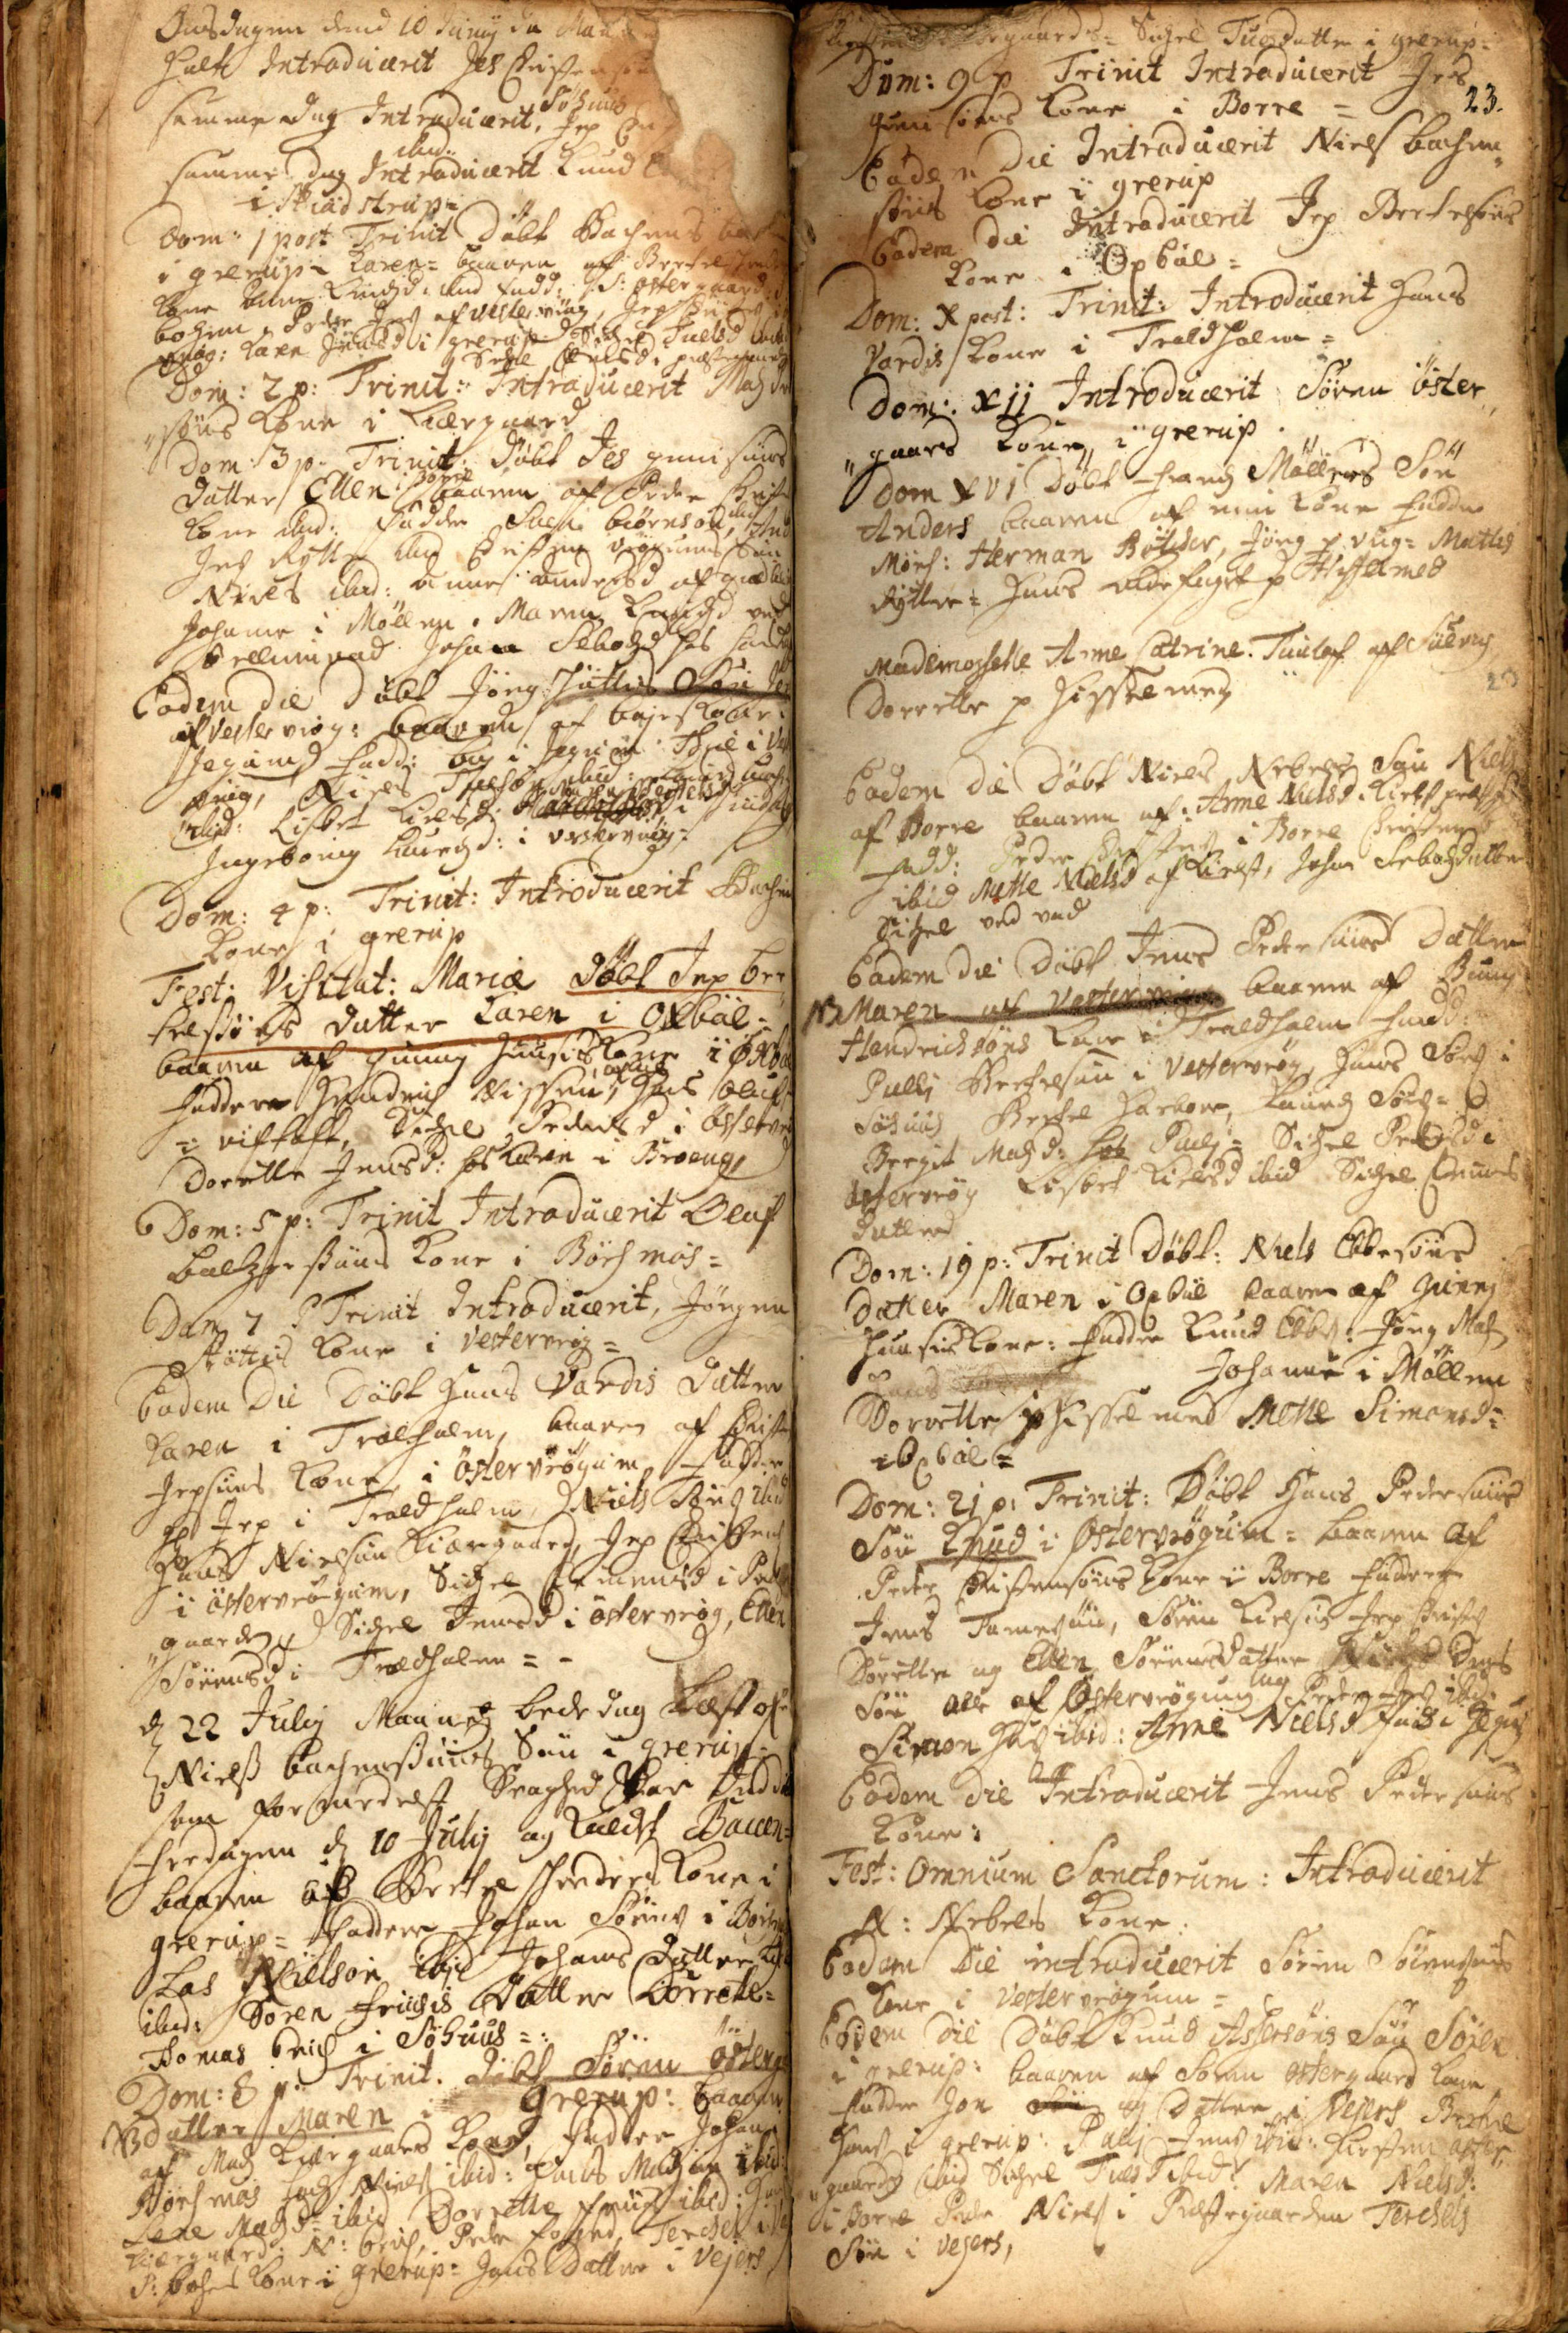Onsdagen dend 10 Junij ## ##
## Introducerit Jes Christen ##
Søhuus
Samme Dag Introduceret Jep ##
ibid:
Samme Dag Introduceiet Knud ##
i Skiødstrup=
Dom: 1 post Trinit Døbt Bachen S##
i Grerup Karen= baaren af Bertel ##
Kone Anne Knudsd ibid Fadd: S. Østergaard##
bachen  Peder Jens af vester vrøg, Jep Christens ##
vrøg: Karen J##d i Grerup: ## Sidsel Tuelsd ##
Sidsel ##elsd i Præstegaard
Dom: 2 p: Trinit. Introducerit Madz Pe
søns Kone i Kiærgaard
Dom: 3 p.Trinit. døbt Jes gunisøns
Datter Ellen i Borre, baaren af Peder Christen
##
Kone ibid: Faddere Sven biørnsøn, And
Jes Rytter ibid Christen Vrøgums Søn
Niels ibid: Anne Andersd af Giedbierg
Johanne i Møllen, Maren Lauridzd ved
Billumvad Johan Sebosd hos ##
Eodem Die døbt Jørg Skøttis Søn Jes
af Vestervrøg: baaren af Bojes Kone ##
Jegum. Fadd: Boj i Jegum, Thue i Vest
vrøg Niels Tuesøn ibid: Lauridz Bach:
##
ibid: Lisbet Kielsd ## i Tudal
Ingeborig Laurdzd: i Vestervrøg:
Dom: 4 p: Trinit: Introducerit Bachens
Kone i Grerup
Fest: Visitat: Mariæ døbt Jep Ber¬
telsøns Datter Karen i Oxbøl=
baaren af Gunnj Juustis Kone i Oxbøl.
Faddere: Hendrich Nissen i Oxbøl, Hans Olufs
i Viftofte, Sidsel Pedersd i Østervrøg
Dorette Jensd: hos Karen i Broeng
Dom: 5 p:Trinit Introducerit Oluf
Balzersøns Kone i Børsmos=
Dom 7 P Trinit Introducerit,  Jørgen
Skøttis Kone i Vestervrøg=
Eodem Die Døbt Hans Vardis Datter
Karen i Troldholm, baaren af Christen
Jepsøns Kone i Østervrøgum - Faddere
gl Jep i Troldholm, Niels ## ibid
Hans Nielsøn Kiærgaard, Jep Christens
i Østervrøgum, Sidzel Clemmensd i Præ
gaarden, Sidzel Jensd i Østervrøg, Ellen
Sørensd i Troldholm= -
d 22 Julij Maanedz Bededag Læst of
Niels Bachensøns Søn i Grerup,
som formedelst Svaghed var Ind##
Fredagen d 10 Julij og kaldet Baccen:
baaren af Bertel Shreders Kone i
Grerup= Faddere Johan Sørens i Bør##
Las Nielsøn ibid, Johans Datter ##
ibid: Søren Friisis Datter Dorrette=
Thomas Brich i Søhuus= :
Dom: 8 p. Trinit. døbt Søren Østerg
s Datter Maren i Grerup: baaren
af Madz Kiærgaards Kone, Fadder Johan i
Børsmos Las Nielsen ibid: Hans Madzøn ibid:
Lene Mdzd= ibid Dorrette Friis ibid: G##
Kiærgaard: N. Brich, Peder Foged, Terchel i V##
##: Bah## Kone i Grerup, Jens Datter i Vejers
23
23
##gaards= Sidzel Tuesdatter i Grerup.
Dom: 9 p Trinit Introducerit Jes
Gunnisøns Kone i Borre=
Eodem Die Introducerit Niels Bachen¬
søns Kone i Grerup
Eoden Die Introducerit Jep Bertelsøns
Kone i Oxbøl=
Dom: X post: Trinit: Introducerit Hans
Vardis Kone i Troldholm=
Dom: XII Introducerit Søren Øster¬
gaards Kone i Grerup.
Dom XVI Døbt Frandz Møllers Søn
Anders baaren af min Kone. Faddere
Møns: Herman Bøtcher, Jørg ##= Mathis
Rytter = Hans Ladefoget p Hisselmed
Mademochelle Anne Catrine. Taulof af Søevig
Dorrette p Hisselmed,
Eodem Die Døbt Niels Nebels Søn Niels
af Borre baaren af: Anne Nielsd. Kielst præsteg
Fadd: Peder Christens i Borre, Christen ##
ibid Mette Nielsd af Kielst, Johan Sebæsdatter
Sidzel ved vad
Eodem Die Døbt Jens Pedersøns Datter
## Maren af Vestervrøg baaren af Gunnj
Hendrichsøns Kone i Troldholm Fadd:
Palle Bertelsøn i Vestervrøg, Hans Sørens i
Søhuus Bertel Harboes, Lauridz Sørens
Bergit Madzd: hos Pallj= Sidzel Pedersd i
Østervrøg Lisbet Kielsd ibid Sidzel Clemmens
datter
Dom: 19 p: Trinit Døbt: Niels Ebbesøns
Datter Maren i Oxbøl baaren af Gunnj
Huusis Kone: Faddere Knud Ebbes:  Jørgen Mads
Hans ##
Johanne i Møllen
Dorrette p Hisselmed, Mette Simonsd:
i Oxbøl=
Dom: 21 p: Trinit: Døbt Hans Pedersøns
Søn Knud i Østervrøgum= baaren af
Peder Christensøns Kone i Borre. Faddere
Jens Tamesøn, Søren Kielsøn Jep Christens
Dorrette og Ellen Sørensdatter Niels ##
Søn alle af Østervrøgum og Peder Jess ibid
Simon H## ibid: Anne Nielsd J## i Jegum
Eodem Die Introducerit Jens Pedersøns
Kone:
Fest. Omnium Sanctorum: Introducerit
N: Nebels Kone:
Eodem Die Introducerit Søren Sørensøns
Kone i Vestervrøgum=
Eodem Die Døbt Knud Assersøns Søn Søren
i Grerup: baaren af Soren Østergaard Kone,
Fadder Jon ## ## Datter i Vejers, Bertel
Hans i Grerup:  Pallj Jens ibid: Kiersten Øster
gaards ibid, Sidzel ## ##: Maren Nielsd:
i Borre Peder Niels i Præstegaarden, Terchels
Søn i Vejers,
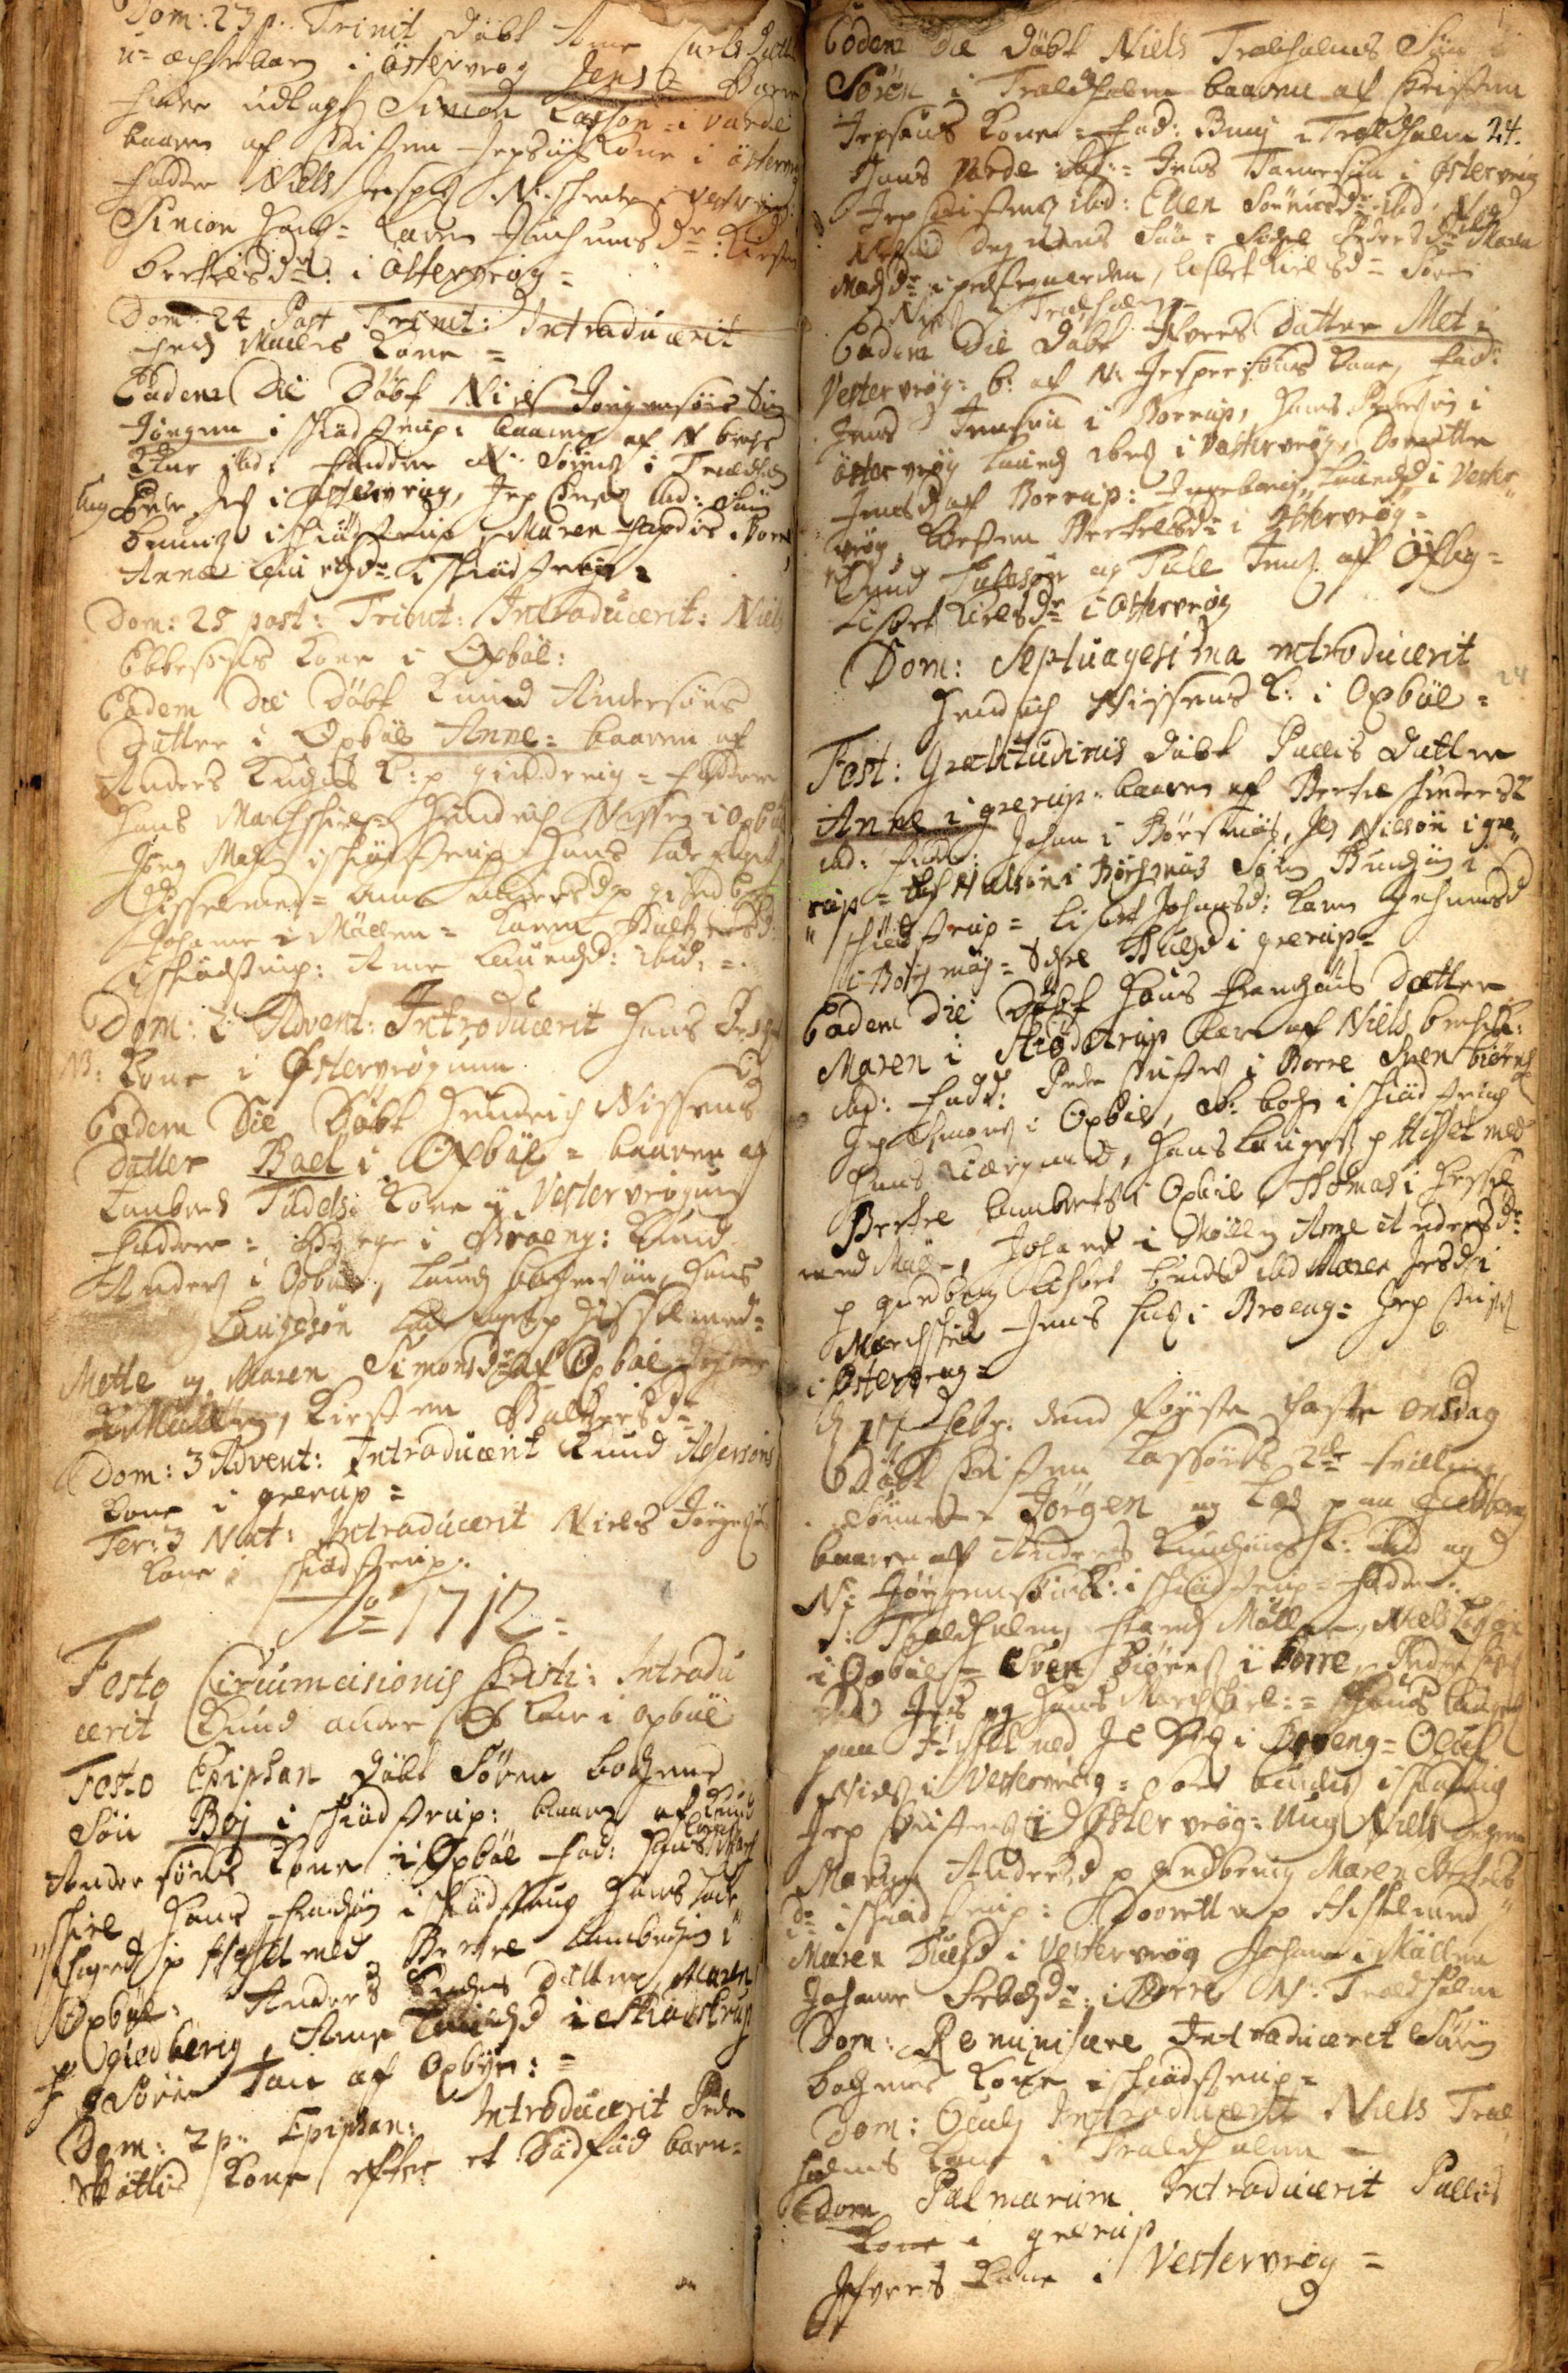Dom: 23 p. Trinit Døbt Anne Carlsdatters
u-ægte barn i Østervrog Jens= Barne
fader udlagt Simon Lassøn=  i Varde
baaren af Christen Jepsøns Kone i østervrog
Fadder Niels Jespers N. Shreder i Vestervrøg.
Simon ##= Karen ##s d'r: Kiersten
Bertelsdr i Østervrøg=
Dom: 24 Post Trinit: Introducerit
## ##s Kone=
Eodem Die Døbt Niels Jørgensøns Søn
Jørgen i Schødstrup: baaren af N Brichs##
Kone ibid: Fadder N. Sørens i Troldh##
Ung Peder Jeps i Østervrøg, Jep Christ ibid, Søren
Bunnis i Schødstrup Maren Fogdis i Borre
Anna Lauritzdr i Schødstrup:
Dom: 25 post: Trinit: Introducerit Niels
Ebbesøns Kone i Oxbøl:
Eodem Die Døbt Knud Andersøns
Datter i Oxbøl Anne: baaren af
Anders Knuds K: p giedderig= Faddere
Hans Marshiel= Hendrich Nissen i Oxbøl
Jørg Mads i Schødstrup Jens Ladefoget
Hisselmed= Anne Andersdr p Giedberg
Johanne i Møllen= Karen Bachens##dr
i Schødstrup: Anne Lauridzd: ibid: = .
Dom: 2 Advent: Introducerit Hans Peders
NB: Kone i Østervrøgum.
Eodem Die Døbt Hendrich Nissens
Datter Boel i Oxbøl=  baaren af
Lanbers Tudels Kone i Vestervrøgum
Faddere: Byrge i Broeng: Knud
Andersen i Oxbøl, Lauridz Bachensøn, Hans
Laugesøn Ladefoget p Hisselmed:
Mette og Maren Simonsdr af Oxbøl Johanne
Terkels##, Kirsten Balthersdr
Dom: 3 Advent: Introducerit Knud Adsersøns
Kone i Grerup=
Fer: 3 Nat: Introducerit Niels Jørgensøns
Kone i Skødstrup:
Ao 1712=
Festo Circumcisionis Christi: Introdu
cerit Knud Anders Kone i Oxbøl
Festo Epiphan døbt Søren Bodsens
Søn Boj i Schiødstrup: baaren af Knud
##
Andersøns Kone i Oxbøl Fad: Hans Mark
skiel, Hans Frandsøn i Schiødstrup, Hans ## Lade¬
foged p Hesselmed Bertel ## i
Oxbøl: Anders ##s Datter, ##
p Giedberig, Anne Lauridsd i Skødstrup
Søren Tais af Oxbye: =
Dom: 2 p: Epiphan: Introducerit Peder
Skøttis Kone efter et dødfød Barn:
24
24
Eodem Die Døbt Niels Trolholms Søn
Søren i Troldholm, baaren af Christen
Jepsøns Kone= Fad: Buni i Troldholm
Hans Varde ibid: Jens Tamesøn i Østervrøg
Jep Christensen ibid: Ellen Sørensdr ibd ##
##.
## Degnens Søn= Sidzel Pedersdr ibd, Maren##
Madzdr i Præstegaarden, Lisbet Kielsd= Søren
Nielsen i Trolholm
Eodem Die Døbt Ifvers Datter Met i
Vestervrøg: b. af N: Jespersøns Kone, Fad:
Jens Jensøn i Borrup, Hans Peders i
Østervrøg Laurids ## i Vestervrøg, Dorrette
Jensd af Borrup: Ingeborig Lauridsd i Vester¬
vrøg, Kirsten Bertelsdr i Østervrøg=
Knud Tulesøn og Tule Jens af Øxby=
Lisbet Kielsdr i Østervrøg
Dom: Septuagesima introducerit
Hendrich Nissens K: i Oxbøl=
Fest: Grat##is døbt Pallis Datter
Anne i Grerup, baaren af Bertel Skreders K
ibd: Fadder: Johan i Børsmøs, Jes Nilsøn i Gre¬
rup= Oluf Nielsøn i Børsmøs, Søren Bundsøn i
Schiødstrup= Lisbet Johansd: Karen Johansd
i Børsmøs= Sidsel Thulsd i Grerup=
Eodem Die Døbt Hans Frandzøns Datter
Maren i Skiødstrup baaren af Niels Brichs## K:
ibd: Fadd: Peder Christens i Borre, Sven Biørns
Jep Christensen## i Oxbøl, S. Bods i Schiødstrup
Hans Kiærgaard, Hans Lauges p Hisselmed
Bertel Lamberts i Oxbøl, Thomas i Hessel
med Mølle, Johanne i Møllen, Anne Andersdr:
p Giedberg Lisbet B##sd ibd, Maren Jesd i
Marschiel - Jens ## i Broeng: Jep Christs
i Østervrog=
D 17 Febr. dend Første Faste Onsdag
Døbt Christen Lassøns 2de tvilling
Sønner Jørgen og Las paa Giedberg
baaren af Anders Knudsøns K: ibd og
N: Jørgensøns K: i Schiødstrup= Fadder
## Troldholm, Frandz Møller, Niels ##
i Oxbøl= Sven Biørns i Borre, Peder Jeps
ibd, Jes og Hans Marskiel: = Hans La##s
paa Hesselmed, Jes ## i Broeng= Oluf
Nielsen i Vestervrøg= Søren Bundes i S##ig
Jep Christens i Østervrøg., Ung Niels Degens##
Maren Andersd p Gedberig, Maren Bertels¬
dr i Schiødstrup, Dorrette p Hesselmed
Maren Tuelsd i Vestervrøg Johanne i Møllen
Johanne Sebædr i Borre, N: Troldholm.
Dom: Reminiscere Introduceret Søren
Bachens Kone i Schiødstrup=
Dom: Oculj Introducerit Niels Trold
holms Kone i Troldholm
Dom Palmarum Introducerit Pallis
Kone i Grerup
Ifvers Kone i Vestervrøg: